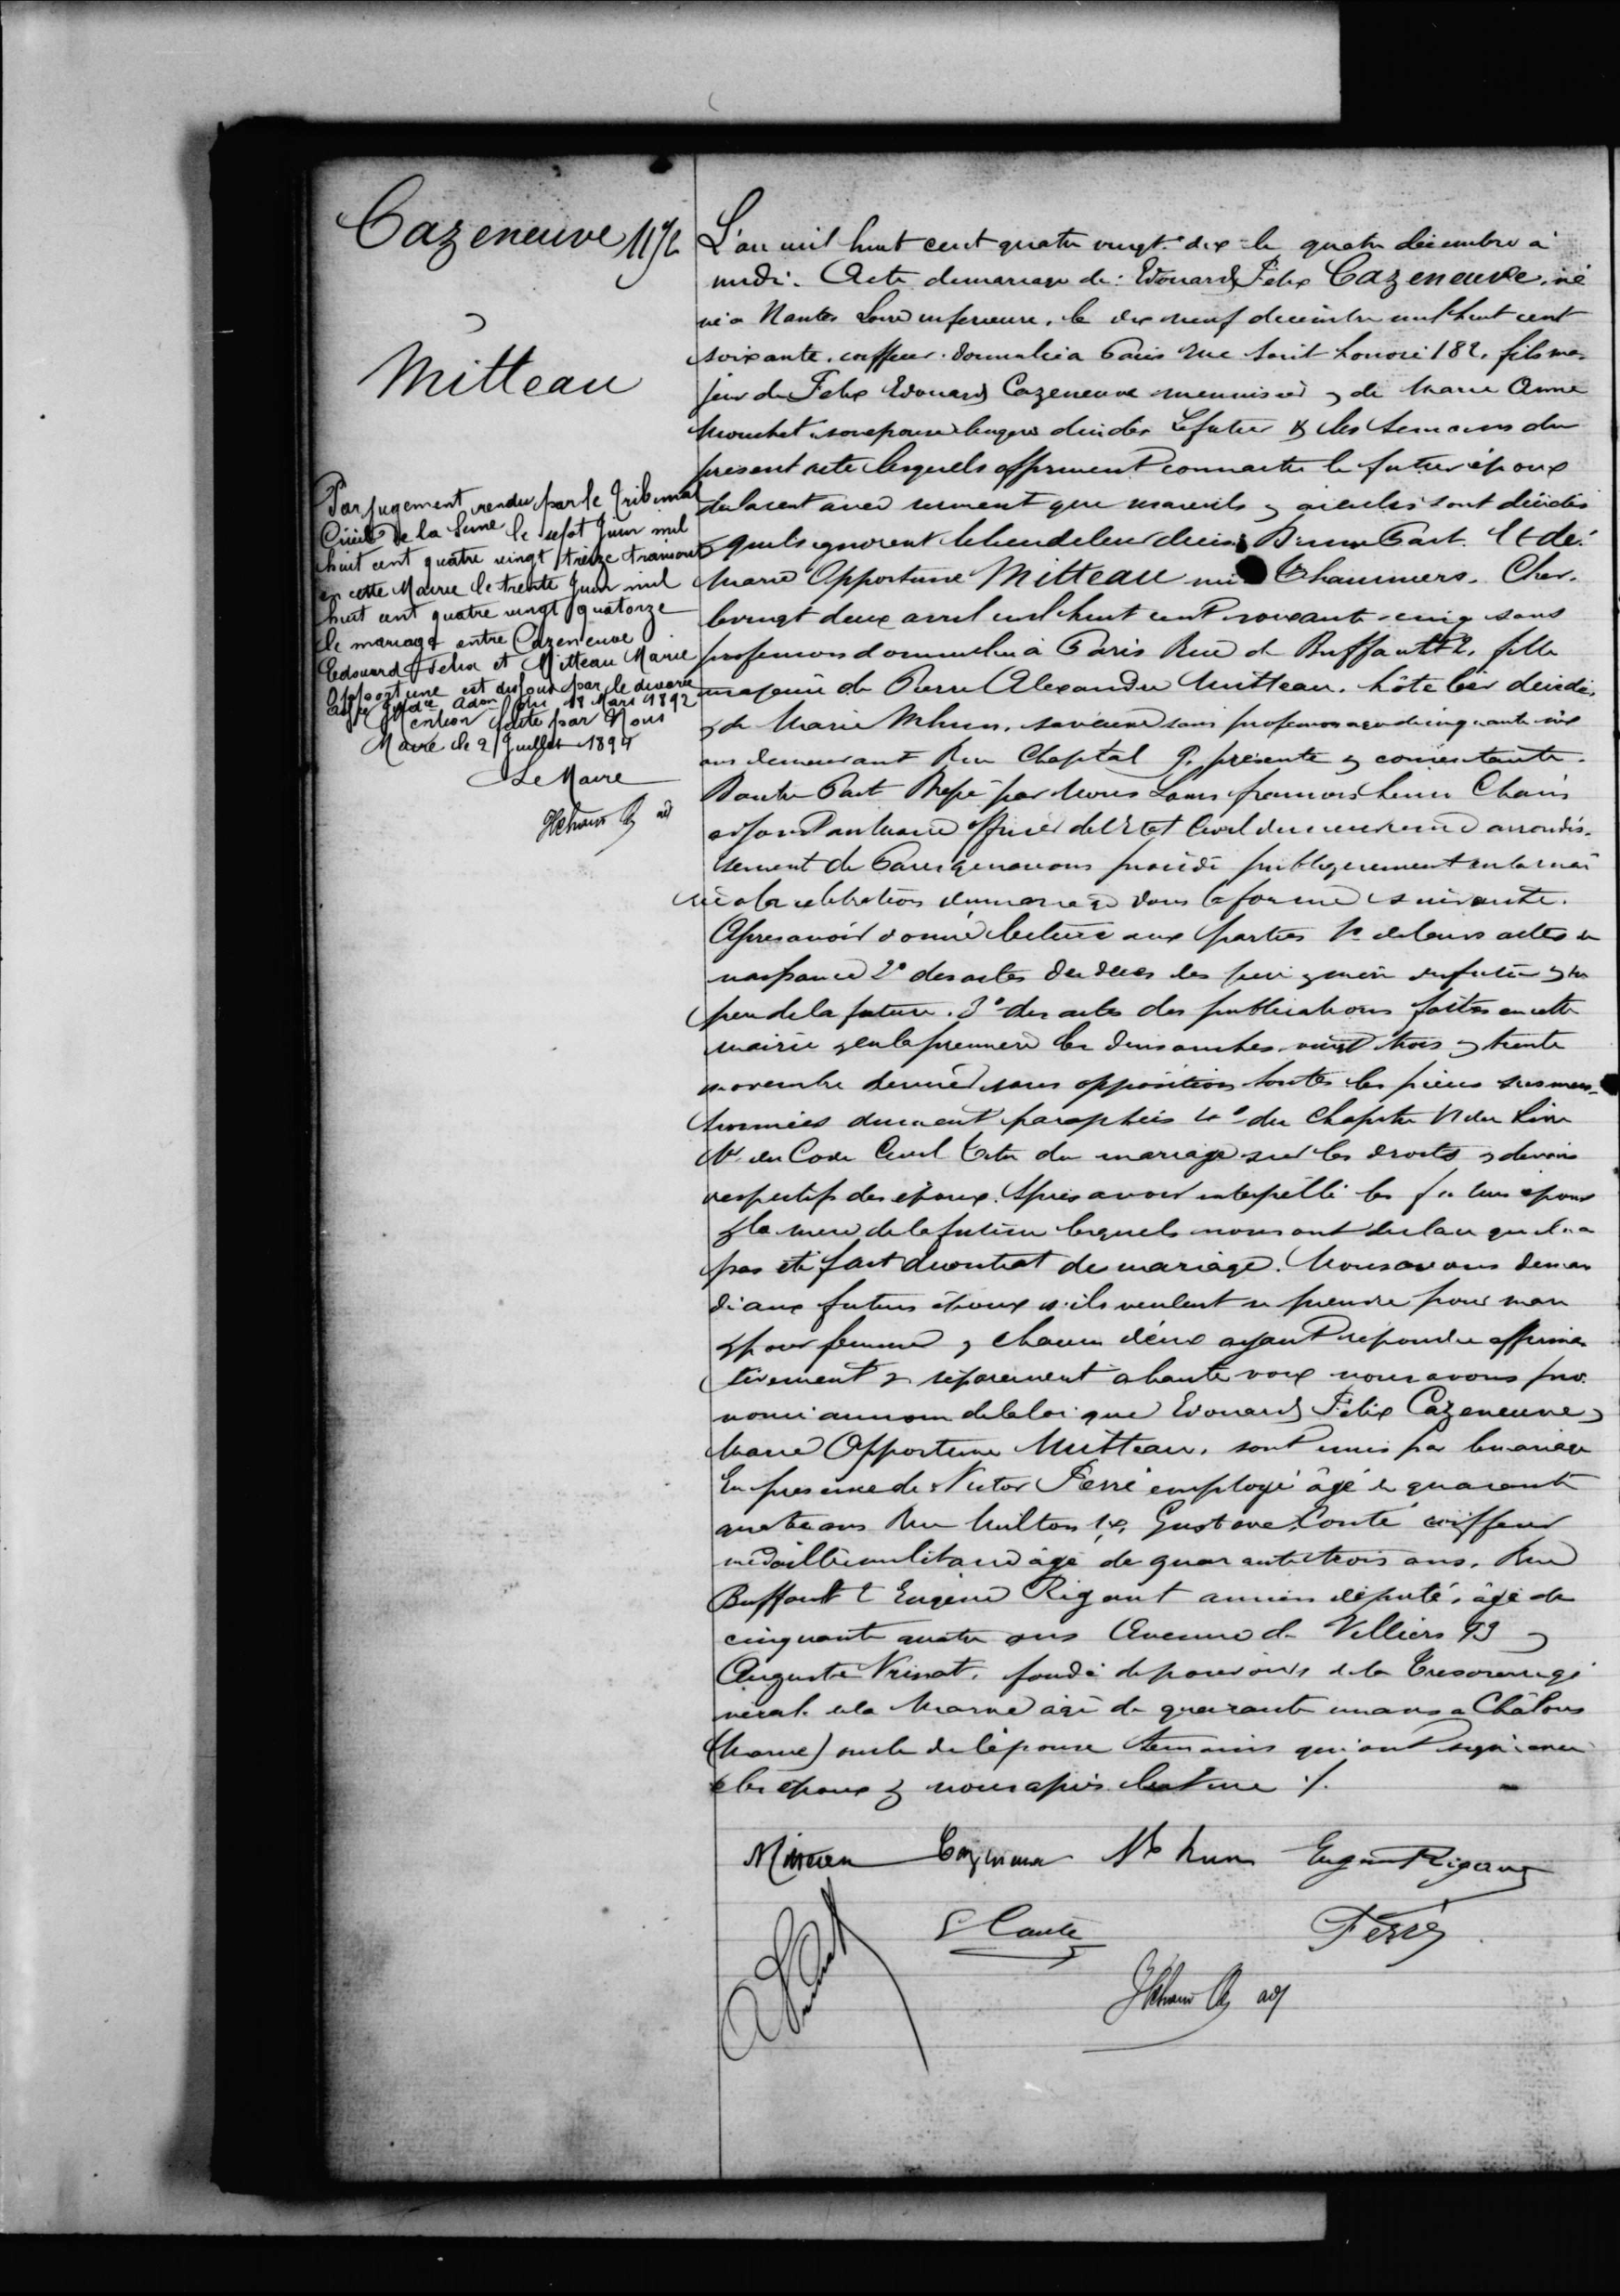Cazeneuve 1172
&
Mitteau
L'an mil huit cent quatre vingt-dix le quatre décembre à
midi. Acte de mariage d'Edouard Félix Cazeneuve, né
né à Nantes Loire inferieure, le dix neuf décembre mil huit cent
soixante. coiffeur. domicilié à Paris rue Saint honoré 182, fils ma-
jeur de Felix Edouard Cazeneuve menuisier, de Marie Anne
Mouchet son epouse lingère decedée. Le futur et les témoins du
présent acte lesquels affirment connaitre les futurs époux
déclarent avec serment que ses aieuils & aieules sont décédés
qu'ils ignorent le lieu de leur décès. D'une Part. Et de
Marie Opportune Mitteau né à Thaumiers. Cher.
le vingt deux avril mil huit cent soixante-cinq sans
profession domicilié à Paris Rue de Buffaut 2, fille
majeure de Pierre Alexandre Mitteau. hôtelier décédé
& de Marie Mhun, sa veuve sans profession agée de cinquante six
ans demeurant Rue Chapital 9, présente & consentante.
D'autre Part Dressé par Nous Louis françois henri Charles
Jean Pantuaud officier de l'état civil du neuvième arrondis
sement de Paris & nous avons procédé publiquement en la mai
rie à la célébration du mariage dans la forme suivante.
Apres avoir donné lecture aux parties 1° de leurs actes de
naissance 2° des actes de décès des père et mère du futur & de
ceu de la future. 3° des actes de publication faites en cette
mairie & en la premiere les dimanches vingt & trente
novembre dernier sans opposition toutes les pieces susmen
tionnées dument paraphées 4° du Chapitre VI du Livre
1er du Code Civil titre du mariage sur les droits & devoirs
respectifs des époux. Après avoir interpellé les futurs époux
la mère de la future lesquels nous ont declaré qu'il n'a
pas été fait de contrat de mariage. Nous avons deman
dé aux futurs époux s'ils veulent se prendre pour mari
et pour femme, chacun d'eux ayant répondu affirma
tivement & séparément à haute voix nous avons pro
noncé aunom delaloi que Edouard Félix Cazeneuve,
Marie Opportune Muitteau, sont unis par le mariage
En presence de Victore Ferré employé âgé de quarante
quatre ans Rue Vuilton 1°, Gustave Conté coiffeur
médaillé militaire âgé de quarante trois ans. Rue
Buffaut 2 Eugéne Rigaut ancien député, âge de
cinquante quatre ans Avenue de Villiers 99,
Auguste Vrinat, fondé de pouvoir de la Trésorerie gé
nérale de la Marne âgé de quarante un ans a Châlons
(Marne) oncle de l'épouse leur ami qui ont signé avec
les époux & nous après lecture./.
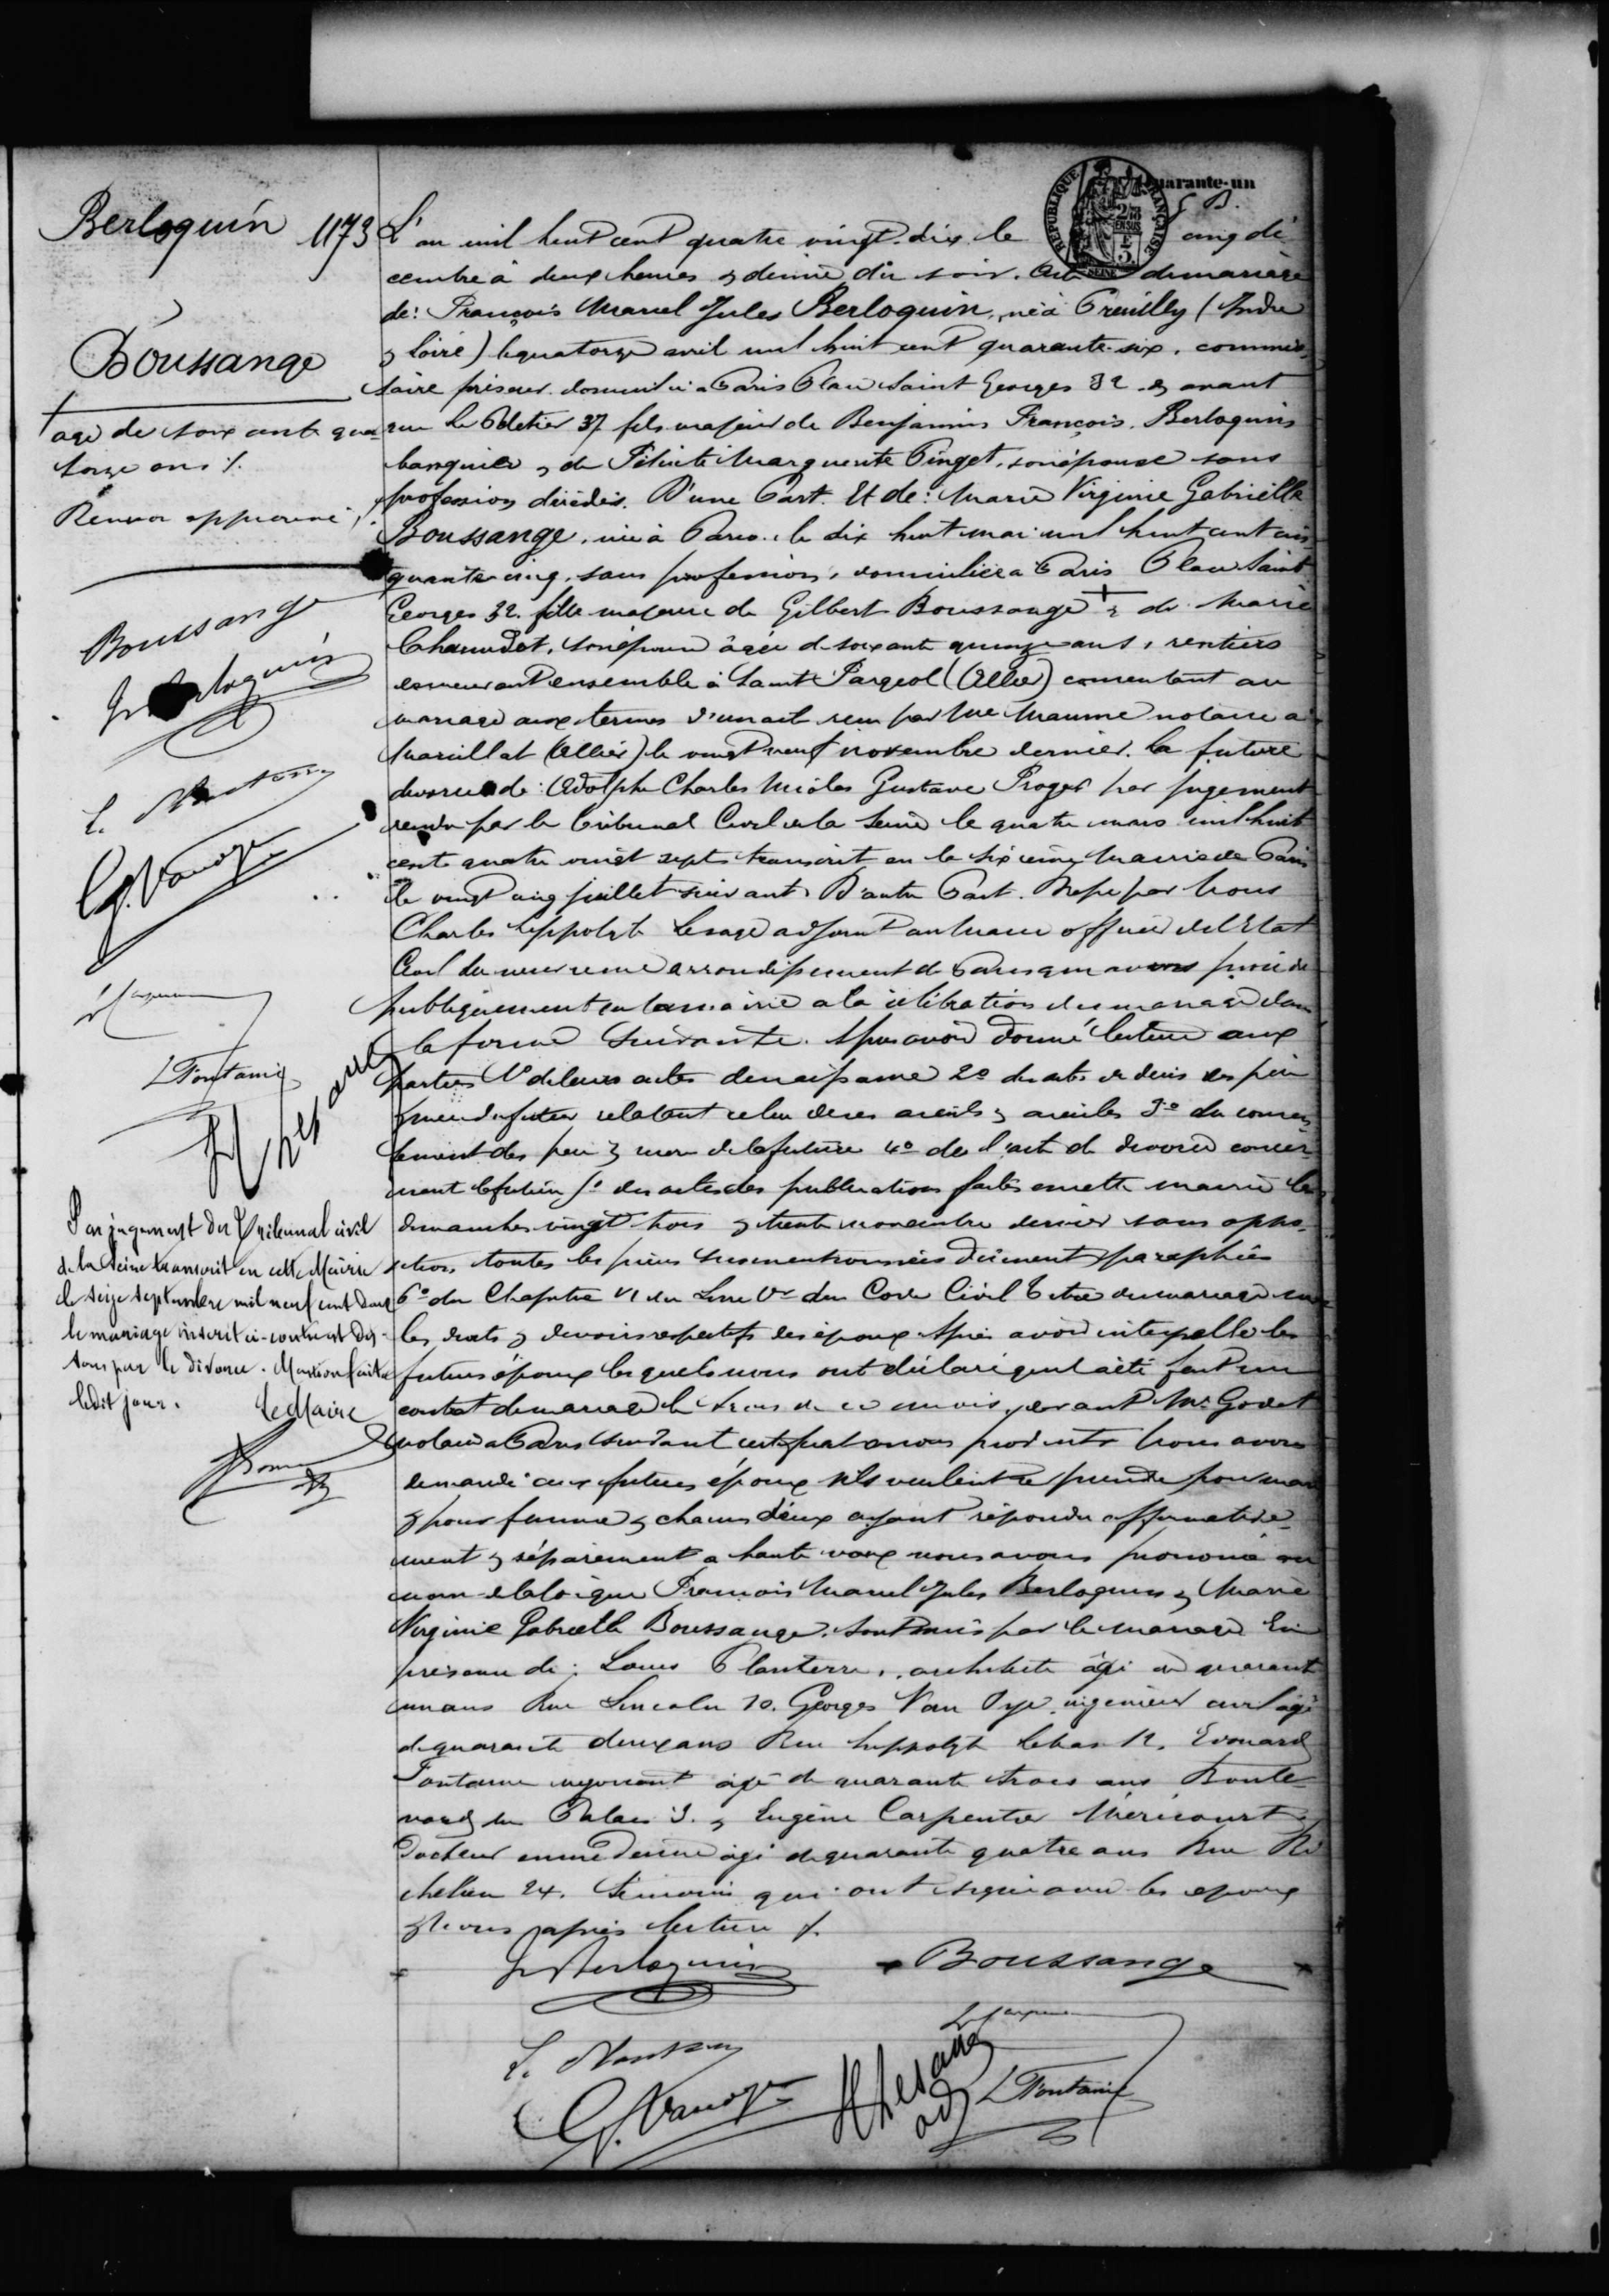Berloquin 1173
&
Boussange
L'an mil huit cent quatre vingt dix le cinq dé
cembre à deux heure & demi du soir. Acte de mariage
de: François Marcel Jules Berloquin, né à a Greuilly (Indre
& loire) le quatorze avril mil huit cent quarante six, commis
saire priseur domicilié à Paris 6 rue Saint Georges 32. avant
rue le Peletier 37 fils majeur de Benjamin François Berloquin
banquier et de Felicité Marguerite Ginget, son épouse sans
profession décédés. D'une Part. Et de: Marie Virginie Gabrielle
Boussange, née à Paris; le dix huit mai mil huit cent cin
quante cinq, sans profession, domiciliée à Paris, Place Saint
Georges 32. fille majeur de Gilbert Boussange & de Marie
Charmadot, sonépouse âgée de soixante quinze ans, rentiers
demeurant ensemble à Saint Fargeot (Allier) consentant au
mariage aux termes d'un acte rendu par Me Maurne notaire à
Mareillat (Allier) le vingt neuf novembre dernier la future
divorce de: Adolphe Charles Nicolas Gustave Roger par jugement
rendu par le Tribunal Civil de la Seine le quatre mars mil huit
cent quatre vingt sept transcrit en la sixième Mairie de Paris
le vingt cinq juillet suivant, D'autre Part. Dresse par Nous
Charles Hippolyte Lesage, adjoint au Maire officier de l'Etat
Civil du neuvieme arrondissement de Paris qui avons procédé
publiquement en la mairie à la célébration du mariage dans
le forme suivante. Après avoir donné lecture aux
parties 1° de leurs actes de naissance 2° des actes de décès des père
et mère du futur relatant celui de ces aieuls & aieules 3° du consente
ment des père et mère de la future 4° de l'acte de divorce concer
nant la futur 5° des actes de publication faite en cette mairie les
dimanches vingt trois & trente novembre dernier sans oppo
sition, toutes les pièces susmentionnées dûment paraphées
6° du Chapitre VI du Livre V du Code Civil Titre du mariage en
les droits et devoirs respectifs des époux après avoir interpellé les
futurs époux lesquels nout ont déclaré quil a été fait un
contrat de mariage le trois de ce mois par devant Me Godet
notaire à Paris rendant certificat avons nous produit nous avons
demandé aux futurs époux s'ils veulent se prendre pour mari
et pour femme chacun d'eux ayant répondu affirmati
vement et séparèment à haute voix nous avons prononcé au
nom de laloi que Francois Marcel Jules Borloguin & Marie
Virginie Gabriella Boussanger, sont unis par le mariage en
présence de: Louis Planterre, architecte, âgé de quarante
un ans, Rue Lincoln 10. Georges Van Pye, ingénieur civil, âgé
de quarante deux ans Rue Hippolyte Lebas 12, Edouard
Fontaine ingénieur âgé de quarante trois ans Boule
vard du Palais 9, Eugène Carpentier Méricourt
Docteur en médecine, âgé de quarante quatre ans Rue Ri
chelieu 24, témoins qui ont signé avec les époux
et nous après lecture./.